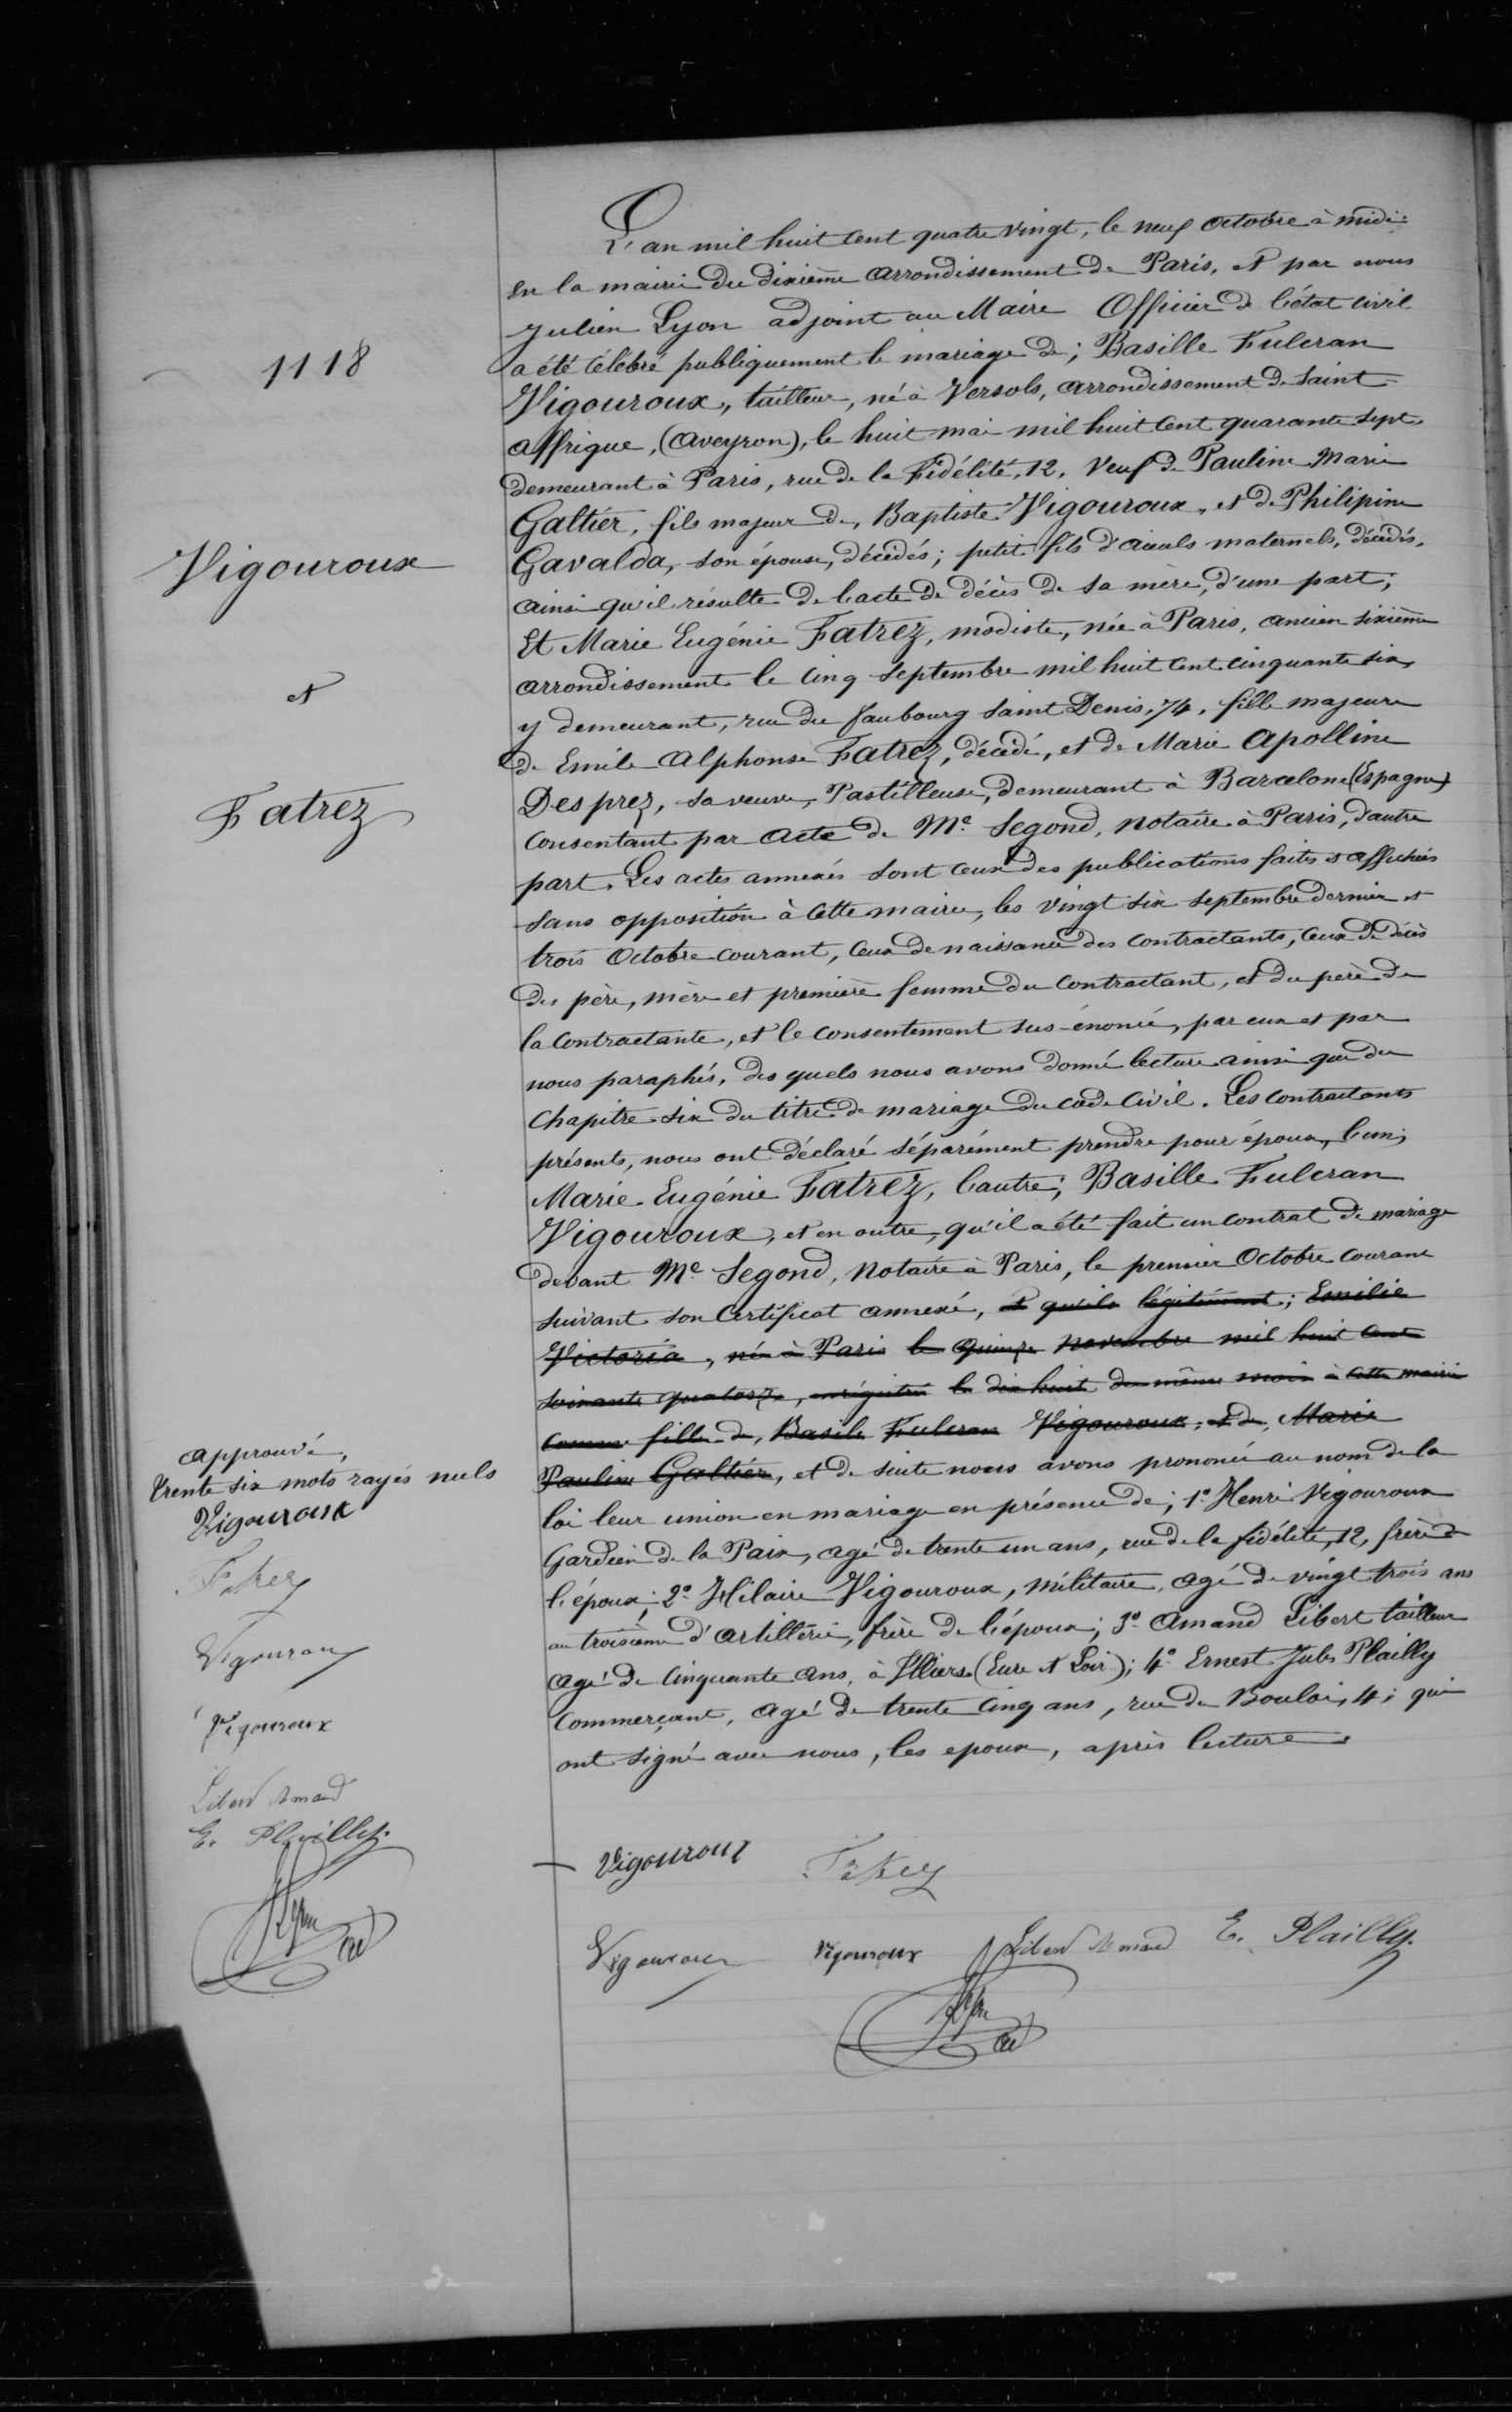1118
Vigouroux
et
Fatrez
L'an mil huit cent quatre vingt, le neuf octobre à midi
En la mairie du dixième arrondissement de Paris et par nous
Julien Lyon adjoint au Maire Officier de l'état civil
a été célébré publiquement le mariage de; Basille Fuleron
Vigouroux, tailleur, né à Versols, arrondissement de saint
affrique, (Aveyron), le huit mai mil huit cent quarante sept
demeurant à Paris, rue de la fidélité, 12, veuf de Paulin Marie
Galtier, fils majeur de, Baptiste Vigouroux, et de Philipine
Gavalda, son épouse, décédés; petit fils d'aieuls maternels, décédés,
ainsi qu'ils résulte de l'acte de décès de sa mère, d'une part;
Et Marie Eugénie Fatrez, modiste, née à Paris, ancien sixième
arrondissement le cinq septembre mil huit cent cinquante six
y demeurant, rue du faubourg saint Denis, 74, fille majeure
d'Emile Alphonse Fatrez décédé, et de MArie APolline
Desprez, sa veuve, Pastilleur, demeurant à Barcelone (Espagne)
consentant par acte de Me Segond, notaire à Paris, d'autre
part. Les actes annexés sont ceux des publications faites et affichées
sans opposition à cette mairie, les vingt six septembre dernier et
trois octobre courant, ceux de naissance des contractants, ceux de décès
des père, mère et première femme du contractant, et du père de
la contractante, et le consentement sus énoncé, par eux et par
nous paraphés, desquels nous avons donné lecture ainsi que du
chapitre six du titre du mariage du code civil. Les contractants
présents, nous ont déclaré séparément prendre pour époux, l'un;
Marie Eugénie Fatrez, l'autre; Basille Fulsan
Vigouroux, et en outre, qu'il a été fait un contrat de mariage
devant Me Segond, notaire à Paris, le premier Octobre courant
suivant son certificat annexé, et qu'ils légitiment; Emilie
Victoria, née à Paris les Quinze Novembre mil huit cent
soixante quatorze, légitimé le dix huit du même mois en cette mairie
comme fille de Basile Fuleron Vigouroux, et de, Marie
Pauline Gaultier, et de suite nous avons prononcé au nom de la
loi leur union en mariage en présence de; 1° Henri Vigouroux
gardien de la Paix, agé de trente un ans, rue de la fidélité, 12, frère de
l'époux; 2° Hilaire Vigouroux, militaire, agé de vingt trois ans
au troisième d'artillerie, frère de l'époux; 3° Armand Libert tailleur
agé de cinquante ans, à Illiers (Eure et Loir); 4° Ernest Jules Plailly
commerçant, agé de trente cinq ans, rue du Bouloi; 4; qui
ont signé avec nous, les epoux, après lecture.
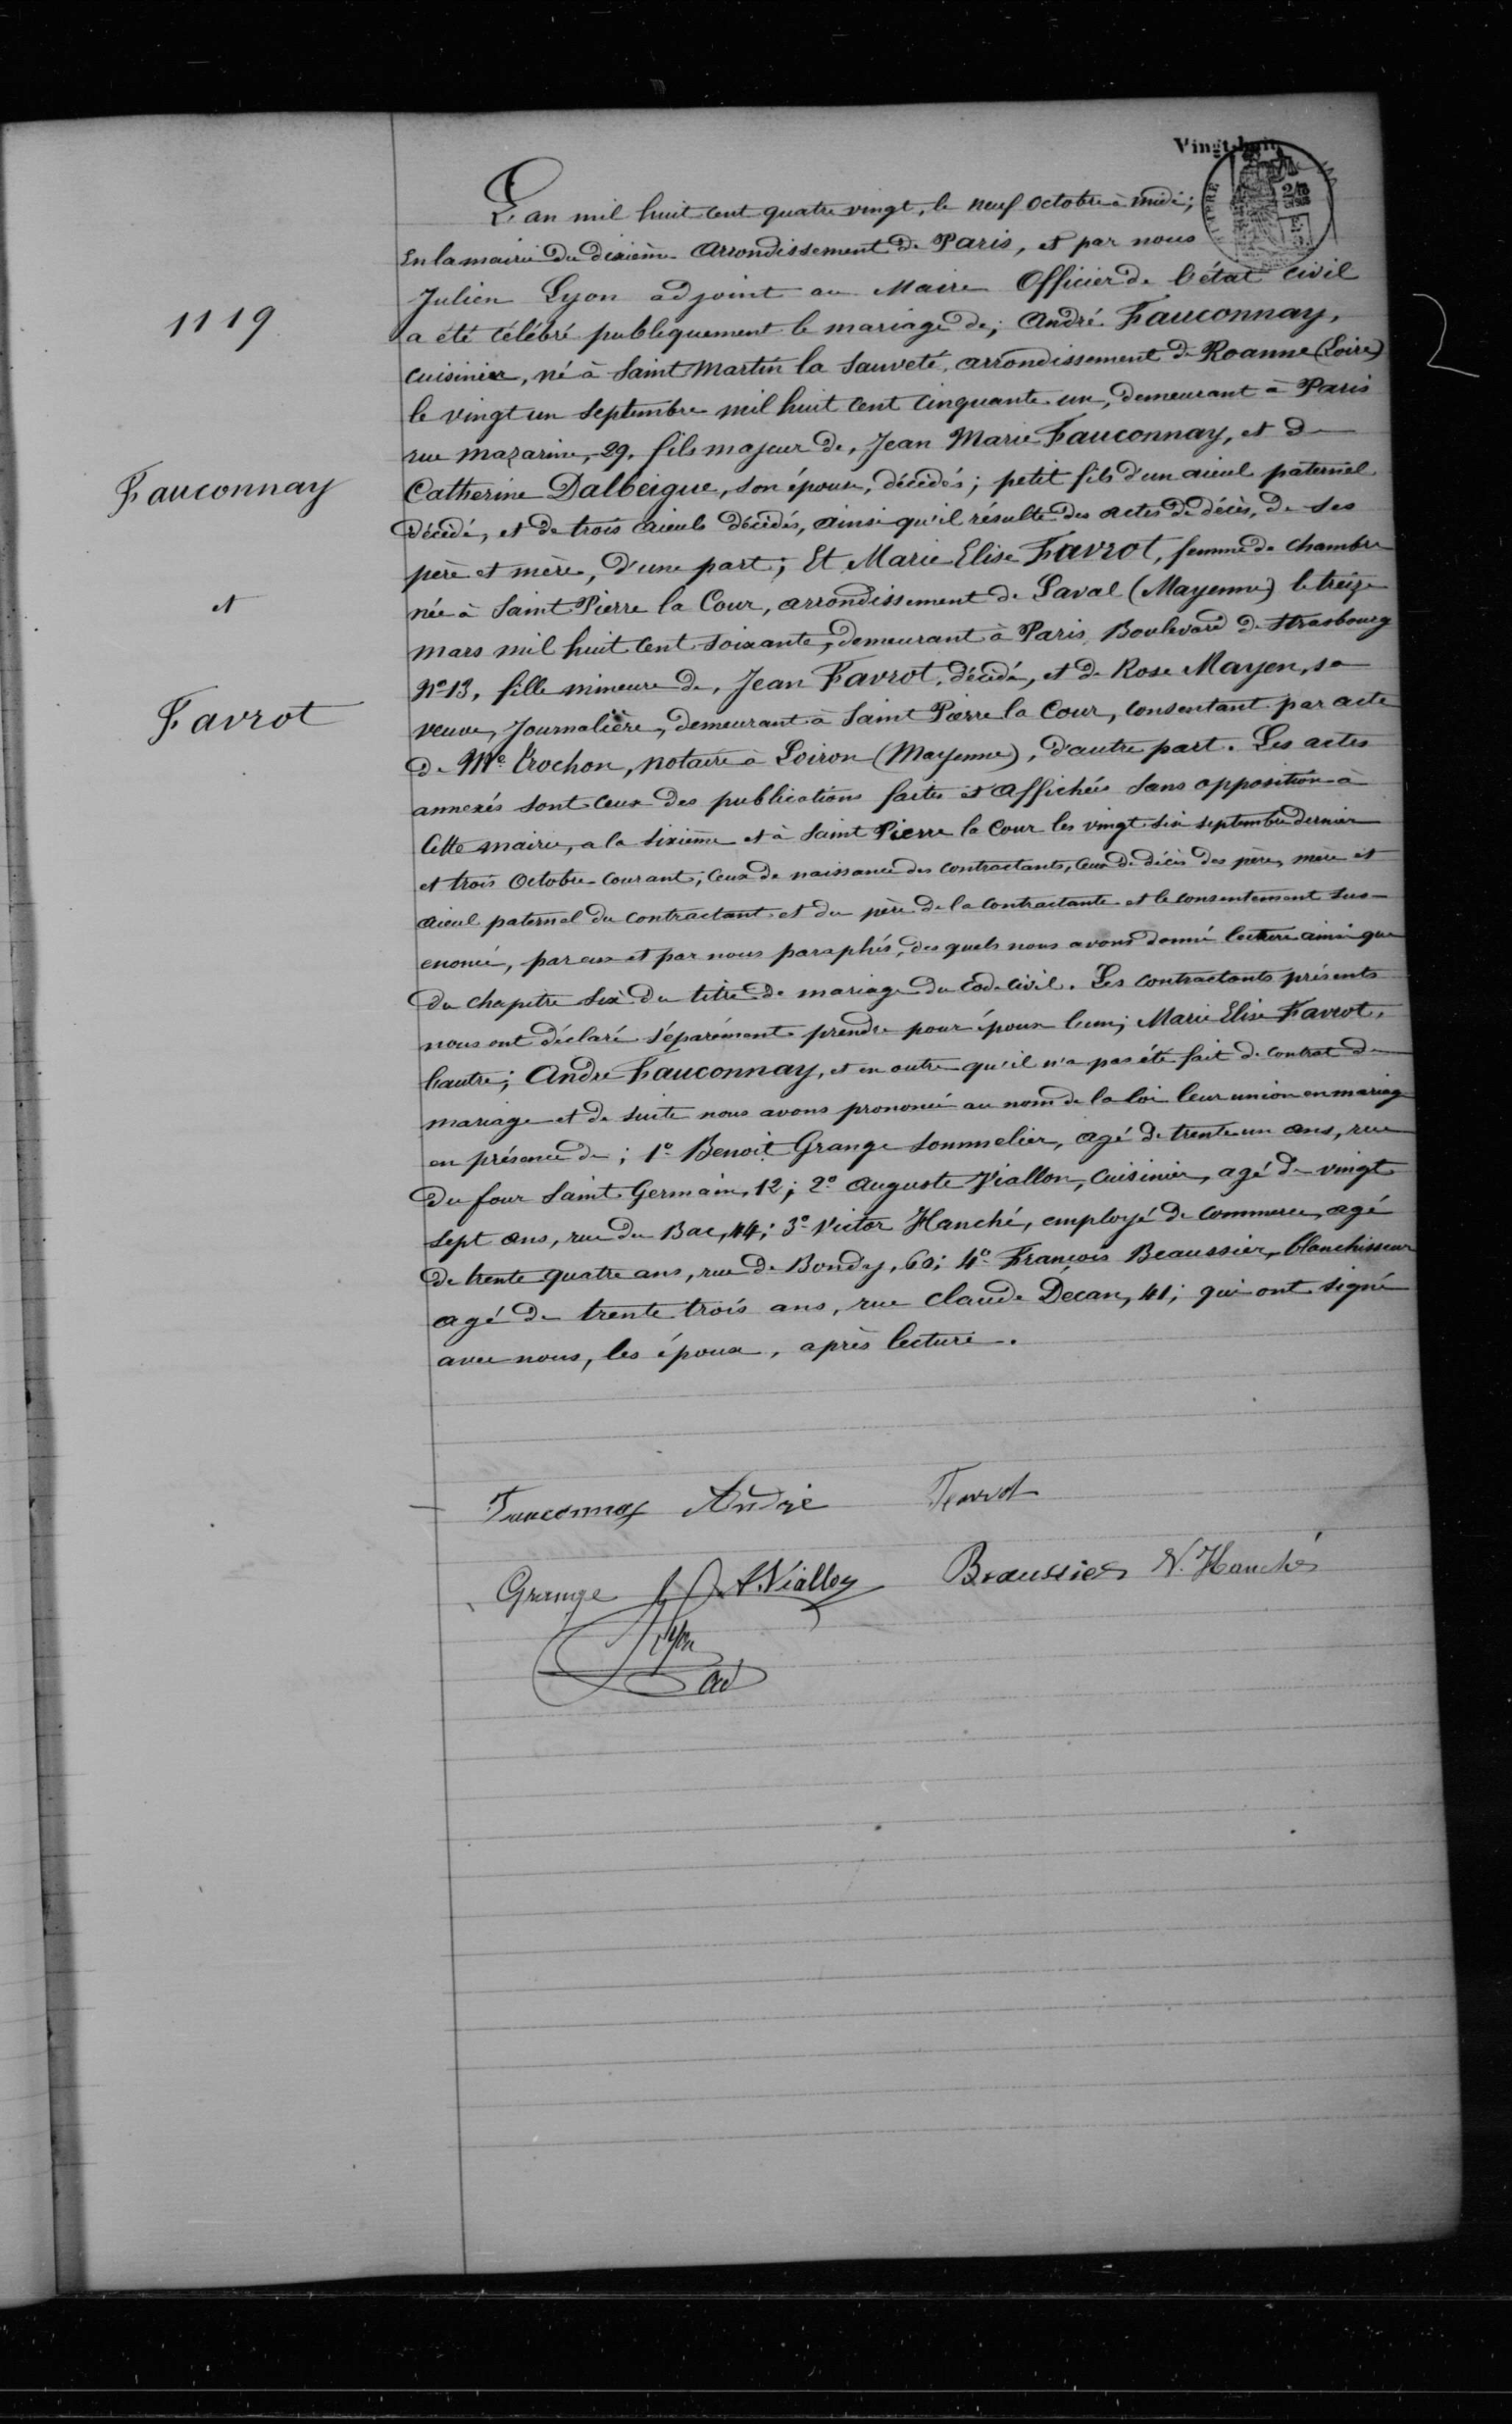1119
Fauconnay
et
Favrot
L'an mil huit cent quatre vingt, le neuf octobre à midi;
En la mairie du dixième arrondissement de Paris, et par nous
Julien Lyon adjoint au Maire Officier de l'état civil
a été célébré publiquement le mariage de; André Fauconnay,
cuisinier, né à Saint Martin la sauvete, arrondissement de Roanne (Loire)
le vingt un septembre mil huit cent cinquante-un, demeurant à Paris
rue Mazarin, 29, fils majeur de, Jean Marie Fauconnay, et de
Catherine Dalbeigue, son épouse, décédés; petit fils d'un aieul paternel
décédé, et de trois aieuls décédés, ainsi qu'il résulte des actes de décès de ses
père et mère, d'une part; Et Marie Elise Favrot, femme de chambre
née à Saint Pierre la Cour, arrondissement de Laval (Mayenne) le treize
mars mil huit cent soixante, demeurant à Paris, Boulevard de Strasbourg
N°13, fille mineure de, Jean Favrot, décédé, et de Rose Mayen, sa
veuve, journalière, demeurant à Saint Pierre la Court, consentant par acte
de Me Brochon, notaire à Loiron (Mayenne), d'autre part. Les actes
annexées sont ceux des publications faites et affichées sans opposition à
cette mairie, a la sixième et à Saint Pierre la Cour les vingt-six septembre dernier
et trois octobre courant, ceux de naissance des contractants, ceux de décès des père, mère et
aieul paternel du contractant et du père de la contractante et consentement sus-
nommé, par eux et par nous paraphés, desquels nous avons donné lecture ainsi que
du chapitre six du titre de mariage du code civil. Les contractants présents
nous ont déclaré séparément prendre pour époux l'un; Marie Elise Favrot,
l'autre; André Fauconnay, et en outre qu'il n'a pas été fait de contrat de
mariage et de suite nous avons prononcé au nom de la loi leur union en mariage
en présence de; 1° Benoit Grange sommelier, agé de trente un ans, rue
du four Saint Germain, 12; 2° Auguste Viallon, cuisinier, agé de vingt
sept as, rue du Bac, 44: 3° Victore Hanché, employé de commerce, agé
de trente quatre ans, rue de Bondy, 60: 4° François Beaussier, blanchisseur
agé de trente trois ans, rue Claude Decan, 41; qui ont signé
avec nous, les époux, après lecture.